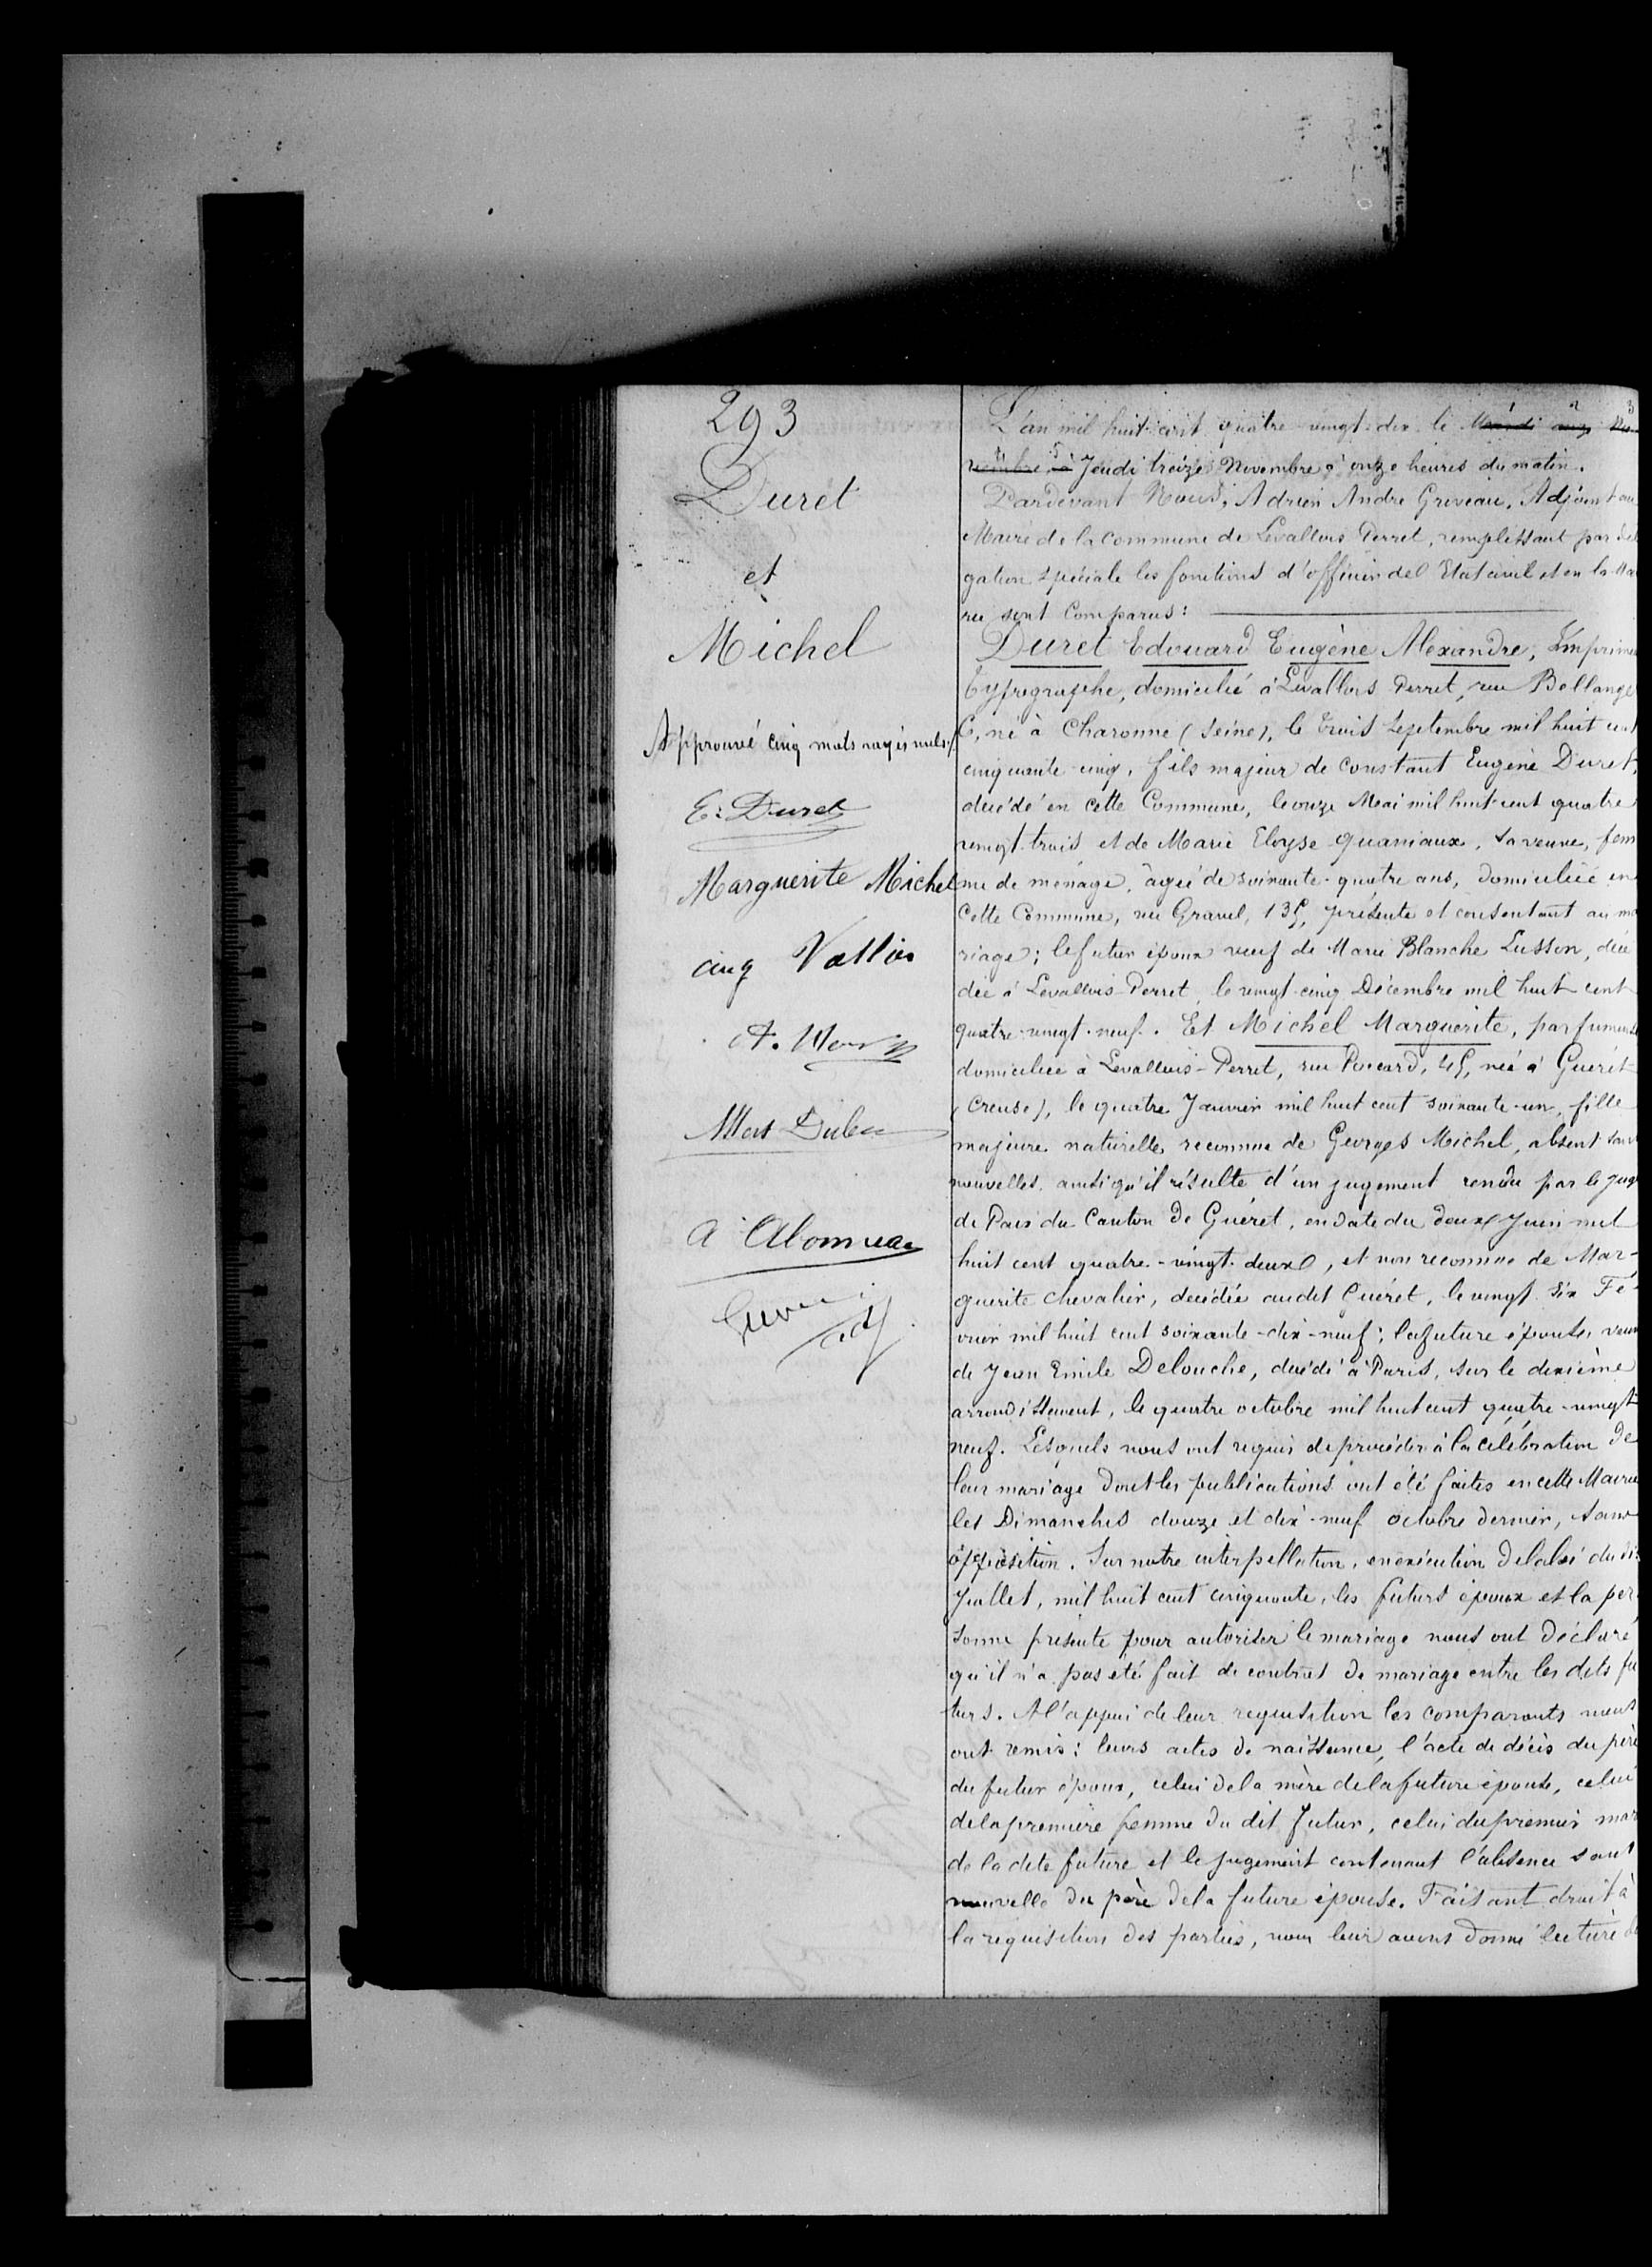L'an mil huit cent quatre vingt dix le Mardi onze heures
novembre à Jeudi treize Novembre à onze heures du matin
Par devant Nous, Adrien Andre Greveau, Adjoint au
Maire de la Commune de Levallois Perret, rempissant par dele
gation spéciale les fonctions d'officier de l'Etat Civil et en la Mai
rie sont Comparus: -
Duret Edouard Eugène Alexandre, Imprimeur
typographe, domicilié à Levallois Perret, rue Bellanger
6, né à Charonne (Seine), le trois septembre mil huit cent
cinquante cinq, fils majeur de Constant Eugène Devret
décédé en cette Commune, le onze Mai mil huit cent quatre
vingt-trois et de Marie Elyse Quaniaux, sa veuve, fem
me de ménage, âgée de soixante-quatre ans, domiciliée en
cette Commune, rue Granel, 135, présente et consentant au ma
riage; le futur époux veuf de mari Blanche Lusson, décé
dée à Levallois-Perret, le vingt-cinq Décembre mil huit cent
quatre-vingt-neuf. Et Michel Marguerite, parfumeur
domiciliée à Levallois-Perret, rue Picard, 45, née à Guerét
(Creuse), le quatre Janvier mil huit cent soixante-un, fille
majeure naturelle reconnue de Georges Michel, absent sans
nouvelles, ainsi qu'il résulte d'un jugement rendu par le juge
de Paris du Canton de Guéret, en date du douze juin mil
huit cent quatre-vingt deux, et non reconnue de Mar-
guerite Chevalier, décédée au dit Guéret, le vingt six Fé-
vrier mil huit cent soixante-dix-neuf; lafuture épouse veuve
de Jean Emile Delouche, décédé à Paris, sur le dixième
arrondissement, le quatre octobre mil huit cent quatre-vingt
neuf. Lesquels nout ont requis de procéder à la célébration de
leur mariage dont les publications ont été faites en cette Mairie
les Dimanches douze et dix-neuf octobre dernier, sans
opposition. Sur notre interpellation, en exécution de la loi du dix
Juillet, mil huit cent cinquante, les futurs époux et la per-
sonne présente pour autoriser le mariage nous ont déclaré
qu'il n'a pas été fait de contrat de mariage entre les dits fu
turs. A l'appui de leur requisition les comparant nous
ont remis: leurs actes de naissance, l'acte de décès du père
du futur époux, celui de la mère delafuture épouse, celui
de la première femme du dit futur, celui du premier mari
de la dite future et le jugement contenant l'absence sans
nouvelle du père de la future épouse. Faisant droit à
La requisition des partis, nous leur avons donné lecture de
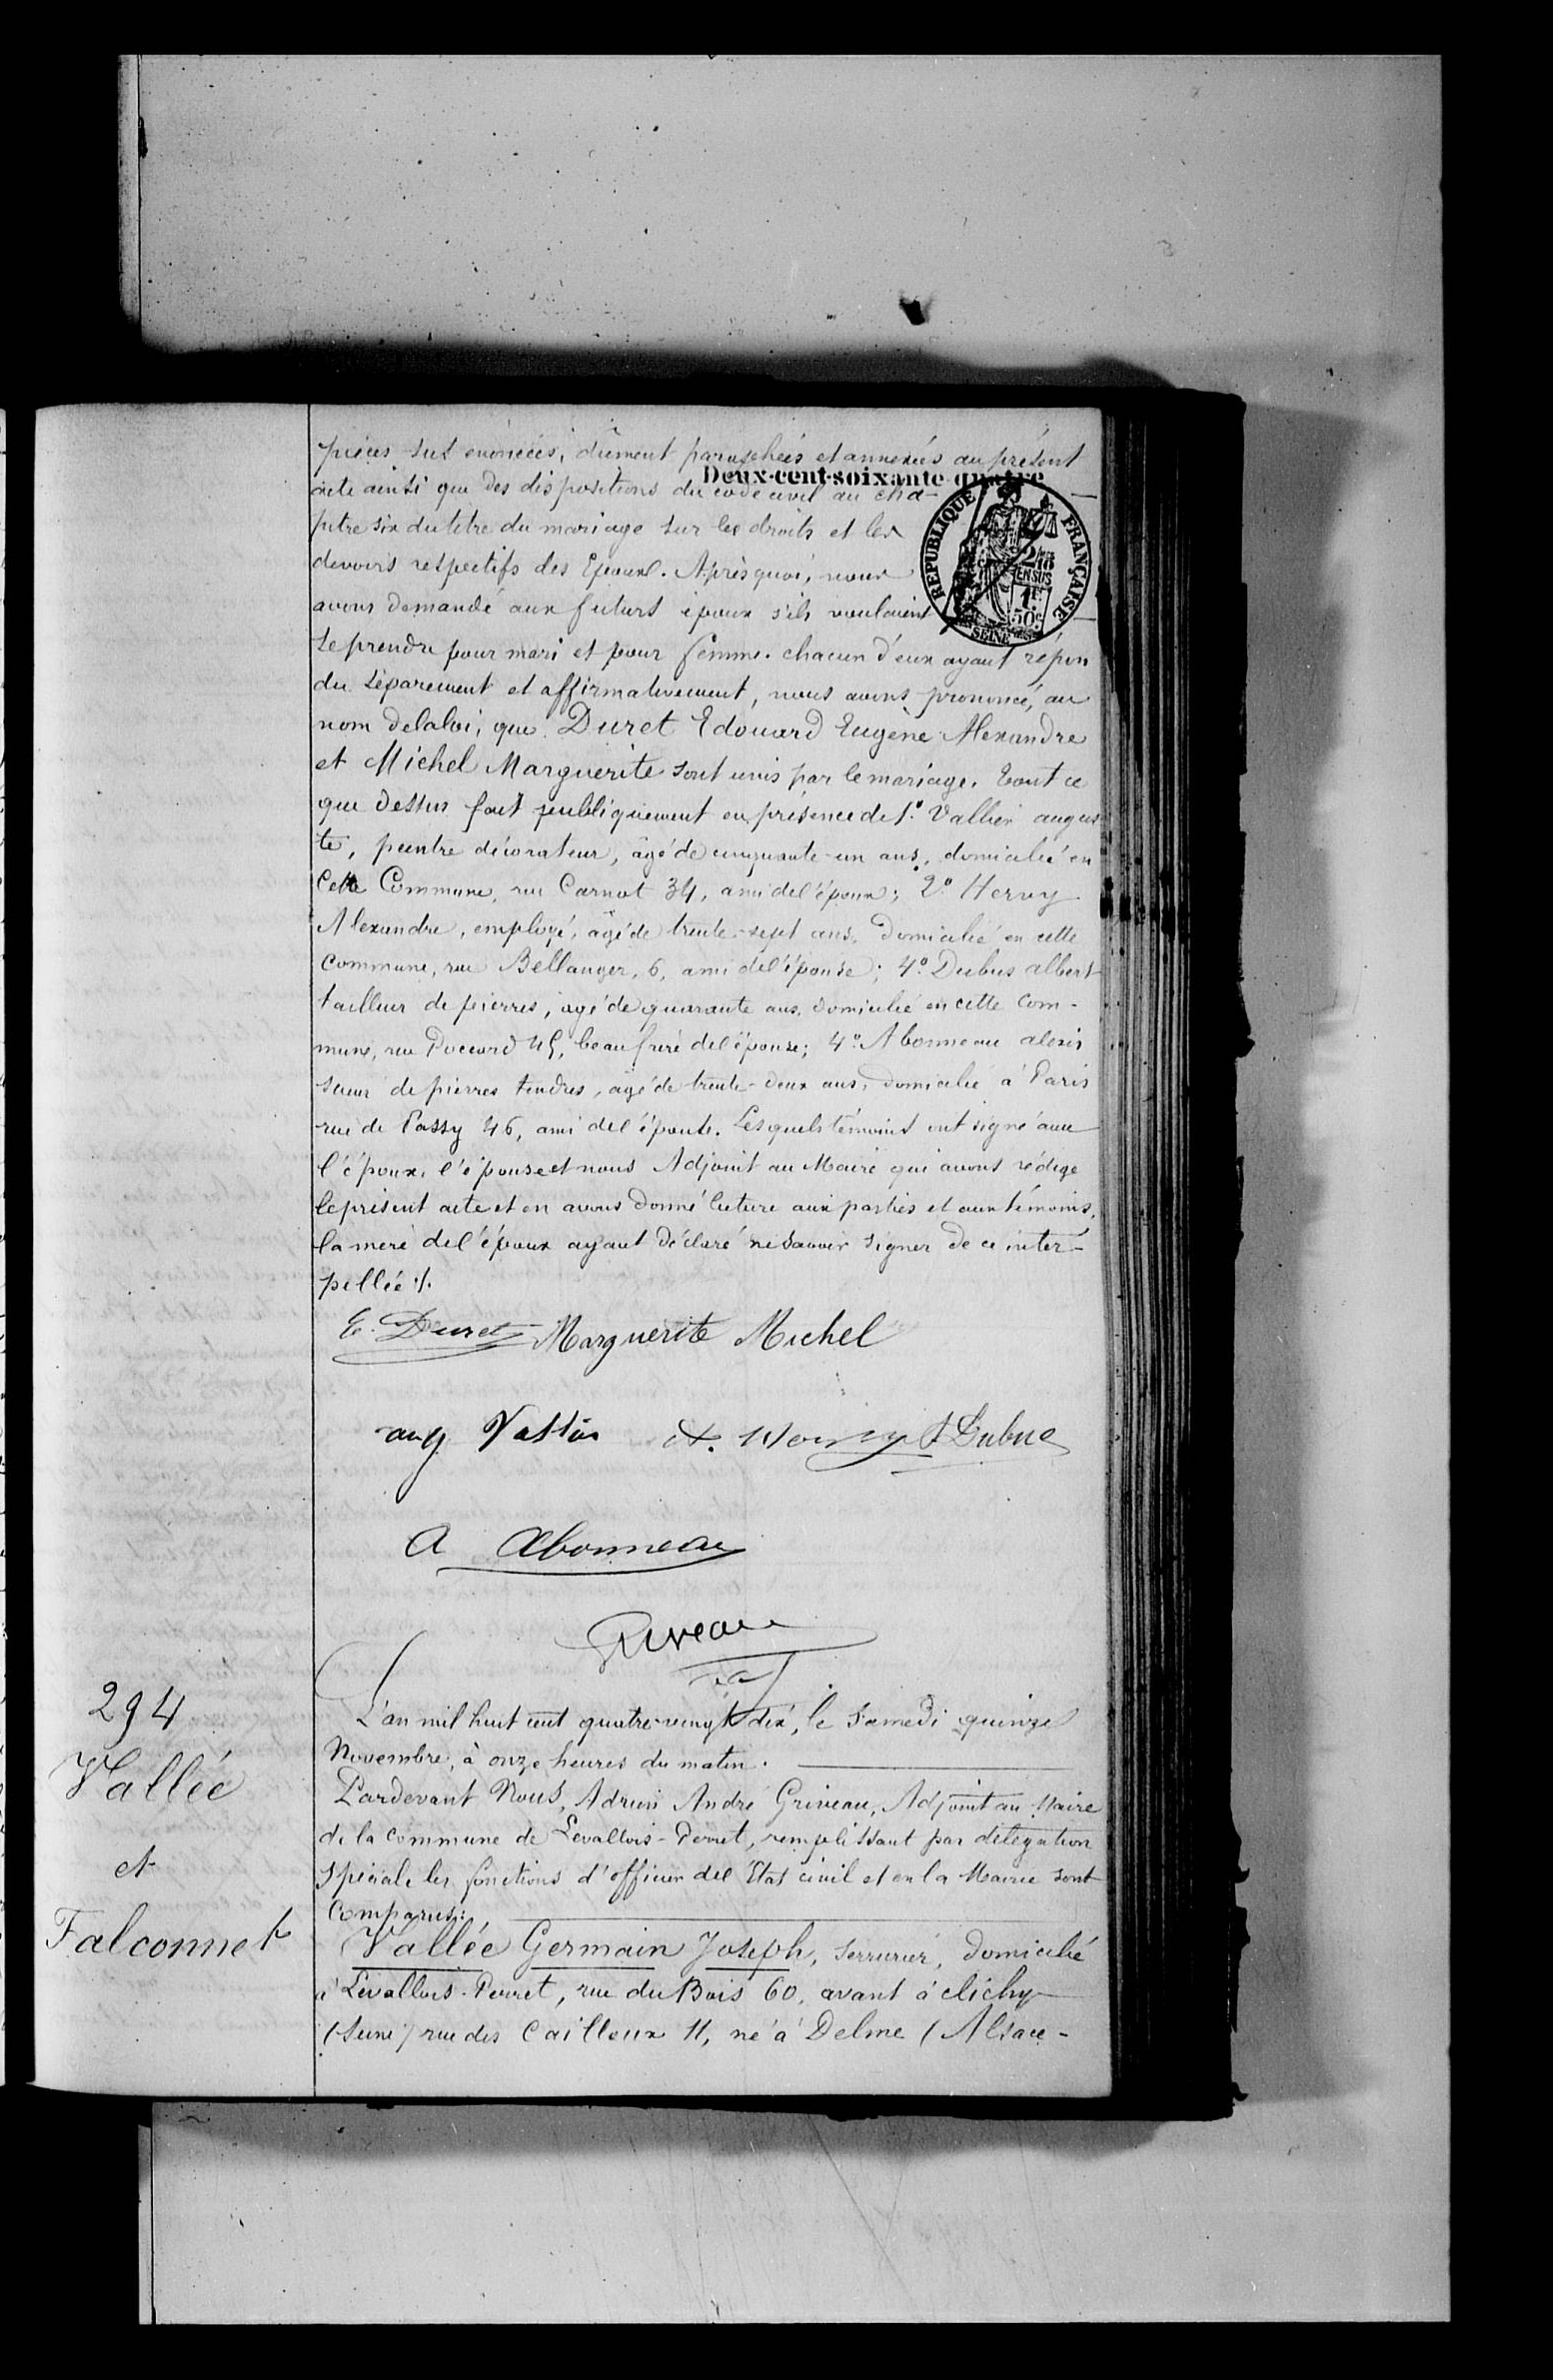pièces sus enoncées, dûment paraphées et annexées au present
acte ainsi que les dispositions du code civil au cha-
pitre six du titre du mariage sur les droits et les
devoirs respectifs des Epoux. Après quoi, nous
avons demandé aux futurs époux s'ils veulent
se prendre pour mari et pour femme chacun d'eux ayant répon
du séparement et affirmativement, nous avons pronnoncé au
nom de la loi, que Duret Edouart Eugène Alexandre
et Michel Marguerite sont unis par le mariage. Tout ce
que dessus font publiquement en présence de 1° Vallien augus
te, peintre décorateur, âgé de cinquante-un ans, domicilié en
cette Commune, rue Carnot 34, ami del'épouse; 2° Henry
Alexandre, employé âgé de trente-sept ans, domicilié en cette
commune, rue Bellanger, 6, ami del'épouse; 4° Dubus Albert
tailleur de pierres, âgé de quarante ans, domicilié en cette com-
mune, rue Piccard 45, beau frère del'épouse; 4°: Abormeau alexis
sieur de pierre tendu, âgé de trente-deux ans, domicilié à Paris
rue de Passy 46, ami del'épouse. Lesquels témoins ont signé avec
l'époux, l'épouse et nous Adjoint au Maire qui avons rédige
léprésent acte et en avons donné lecture aux parties et aux témoins,
la mère del'époux ayant déclaré nesavoir signer de ce inter-
pellée./.
294
Vallée
et
Falconnet
L'an mil huit cent quatre-vingt dix, le samedi quinze
novembre à onze heures du matin.-
Pardevant Nous, Adrien André Griveau, Adjoint au Maire
de la Commune de Levallois-Perret, remplissant par délégation
spéciale les fonction d'officier del'Etat Civil et en la Mairie sont
comparus :-
Vallée Germain Joseph, serrurier, domicilié
à Levallois-Perret, rue du Bois 60, avant à clichy-
(Seine) rue des Cailloux 11, né à Delme (Alsace-